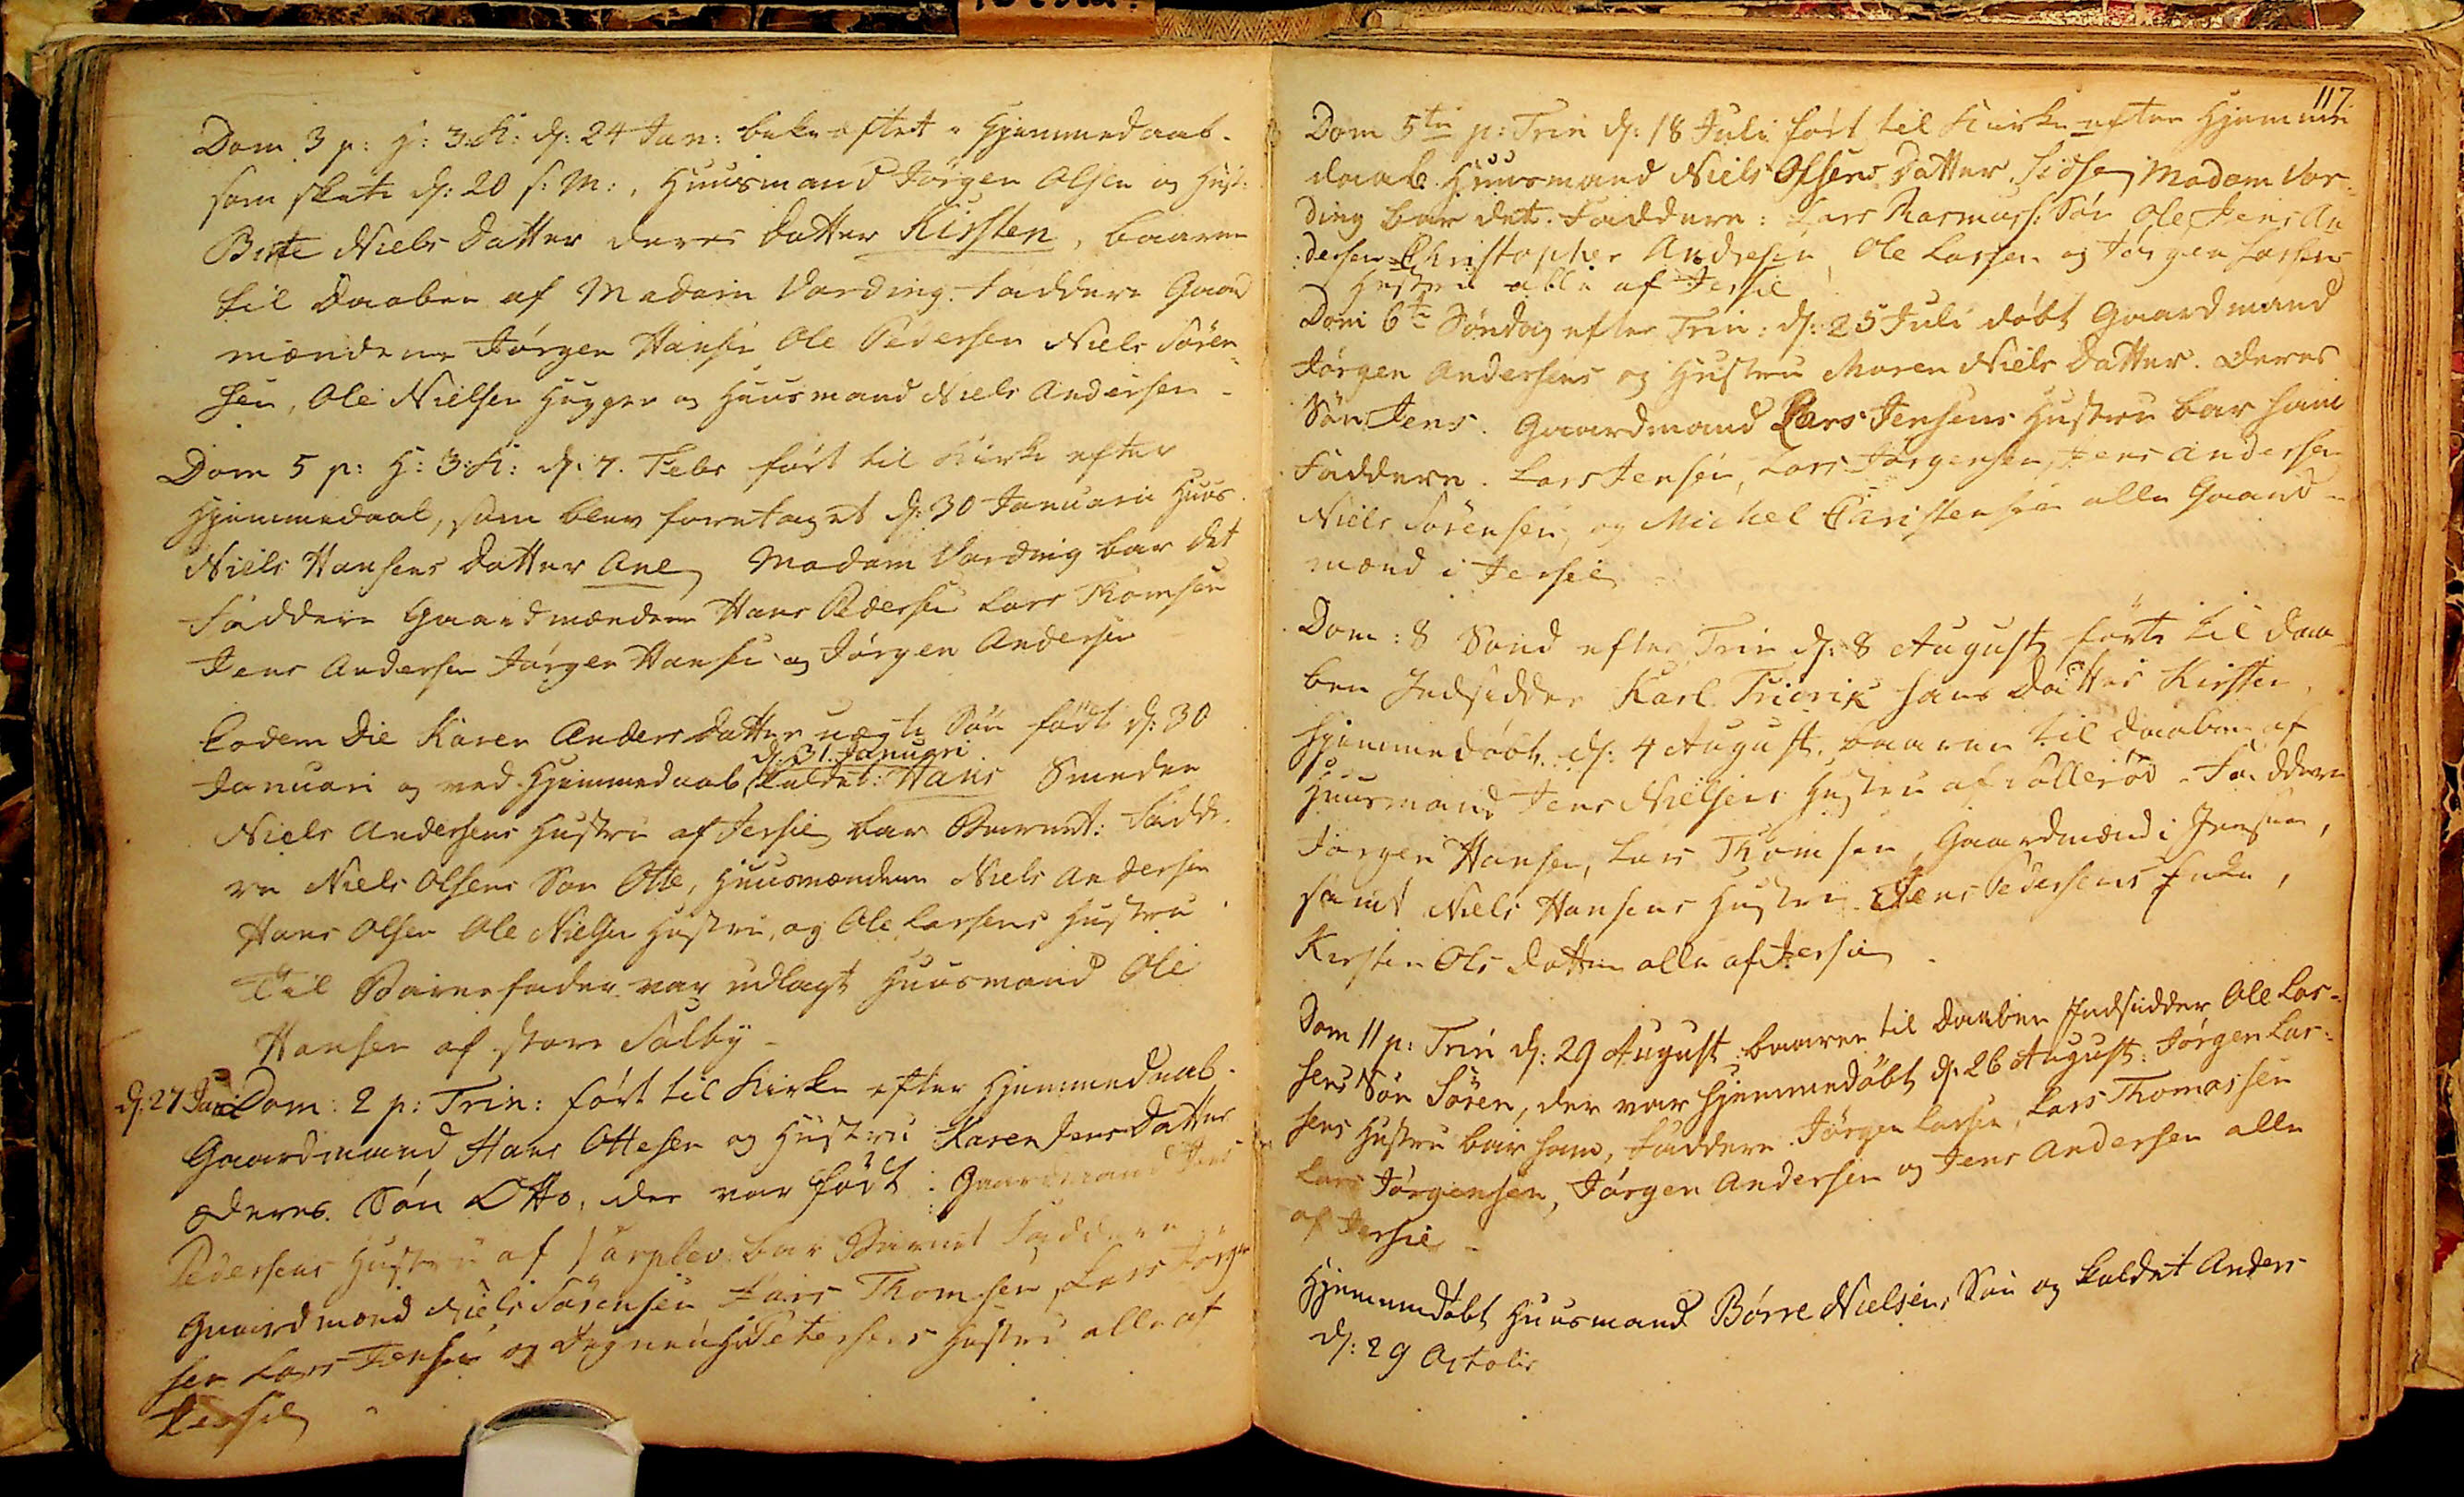Dom. 3 p: H: 3: K: d: 24 Jan: bekræftet e Hjemmedaab,
som skete d: 20 s: m:, Huusmand Jørgen Olsen og Hust:
Berte Niels Datter deres Datter Kirsten, baaren
til Daaben af Madam Vording. Faddere Gaard
mændene Jørgen Hansen Ole Pedersen Niels Søren¬
sen, Ole Nielsen Hugger og Huusmand Niels Andersen.
Dom 5 p: H: 3: K: d: 7. Febr ført til Kirke efter
Hjemmedaab, som blev foretaget d: 30 Januari Huus.
Niels Hansens Datter Ane Madam Vording bar det
Faddere Gaardmændene Hans Pedersen Lars Thomsen
Jens Andersen Jørgen Hansen og Jørgen Andersen
Eodem Die Karen Anders Datter uægte Søn født d: 30
d: 31. Januari
Januari og ved. Hjemmedaab kaldet: Hans Smeden
Niels Andersens Hustru af Jersie bar Barnet: Fadde¬
re Niels Olsens Søn Otte, Huusmændene Niels Andersen
Hans Olsen Ole Nielsens Hustru, og Ole Larsens Hustru
Til Barnefader var udlagt Huusmand Ole
Hansen af Store Salby
d: 27 Jun: Dom: 2 p: trin: ført til Kirke efter Hjemmedaab
Gaardmand Hans Ottesen og Hustru Karen Jens Datter
deres Søn Otto, der var født. Gaardmand Jens
Pedersens Hustru af Varplev: bar Barnet Faddere
Gaardmand Niels Sørensen Hans Thomsen, Lars Jørgen
sen. Lars Jensen og Degnen Hr Petersens Hustru alle af
Jersie.
117.
Dom 5te p: Trin d: 18 Juli ført til Kirke efter Hjemme
daab Huusmand Niels Olsens Datter Sidse Madam Vor¬
ding bar det. Faddere: Lars Rasmuss: Søn Ole, Jens An
dersen Christopher Andresen, Ole Larsen og Jørgen Larsens
Hustrue alle af Jersie
Dom 6te Søndag efter Trin: d: 25 Juli døbt Gaardmand
Jørgen Andersens og Hustru Maren Niels Datter. Deres
Søn Jens. Gaardmand Lars Jensens Hustru bar ham
Faddere. Lars Jensen, Lars Jørgensen, Jens Andersen
Niels Sørensen, og Michel Christensen alle Gaard¬
mænd i Jersie
Dom: 8 Sønd efter Trin d: 8 August ført til Daa¬
ben Indsidder Karl Fridrik Hans Datter Kirsten,
hjemmedøbt d: 4 August, baaren til Daaben af
Huusmand Jens Nielsens Hustru af Sollerød. Faddere
Jørgen Hansen, Lars Thomsen Gaardmænd i Jersie,
samt Niels Hansens Hustru. Jens Pedersens Enke,
Kirsten Ols Datter alle af Jersie.
Dom 11 p: Trin d: 29 August baaren til Daaben Indsidder Ole Lar¬
sens Søn Søren, der var hjemmedøbt d: 26 August: Jørgen Lar¬
sens Hustru bar ham, Faddere. Jørgen Larsen, Lars Thomassen
Lars Jørgensen, Jørgen Andersen og Jens Andersen alle
af Jersie 
Hjemmedøbt Huusmand Børre Nielsens Søn og kaldet Anders
d: 29 Octobr 
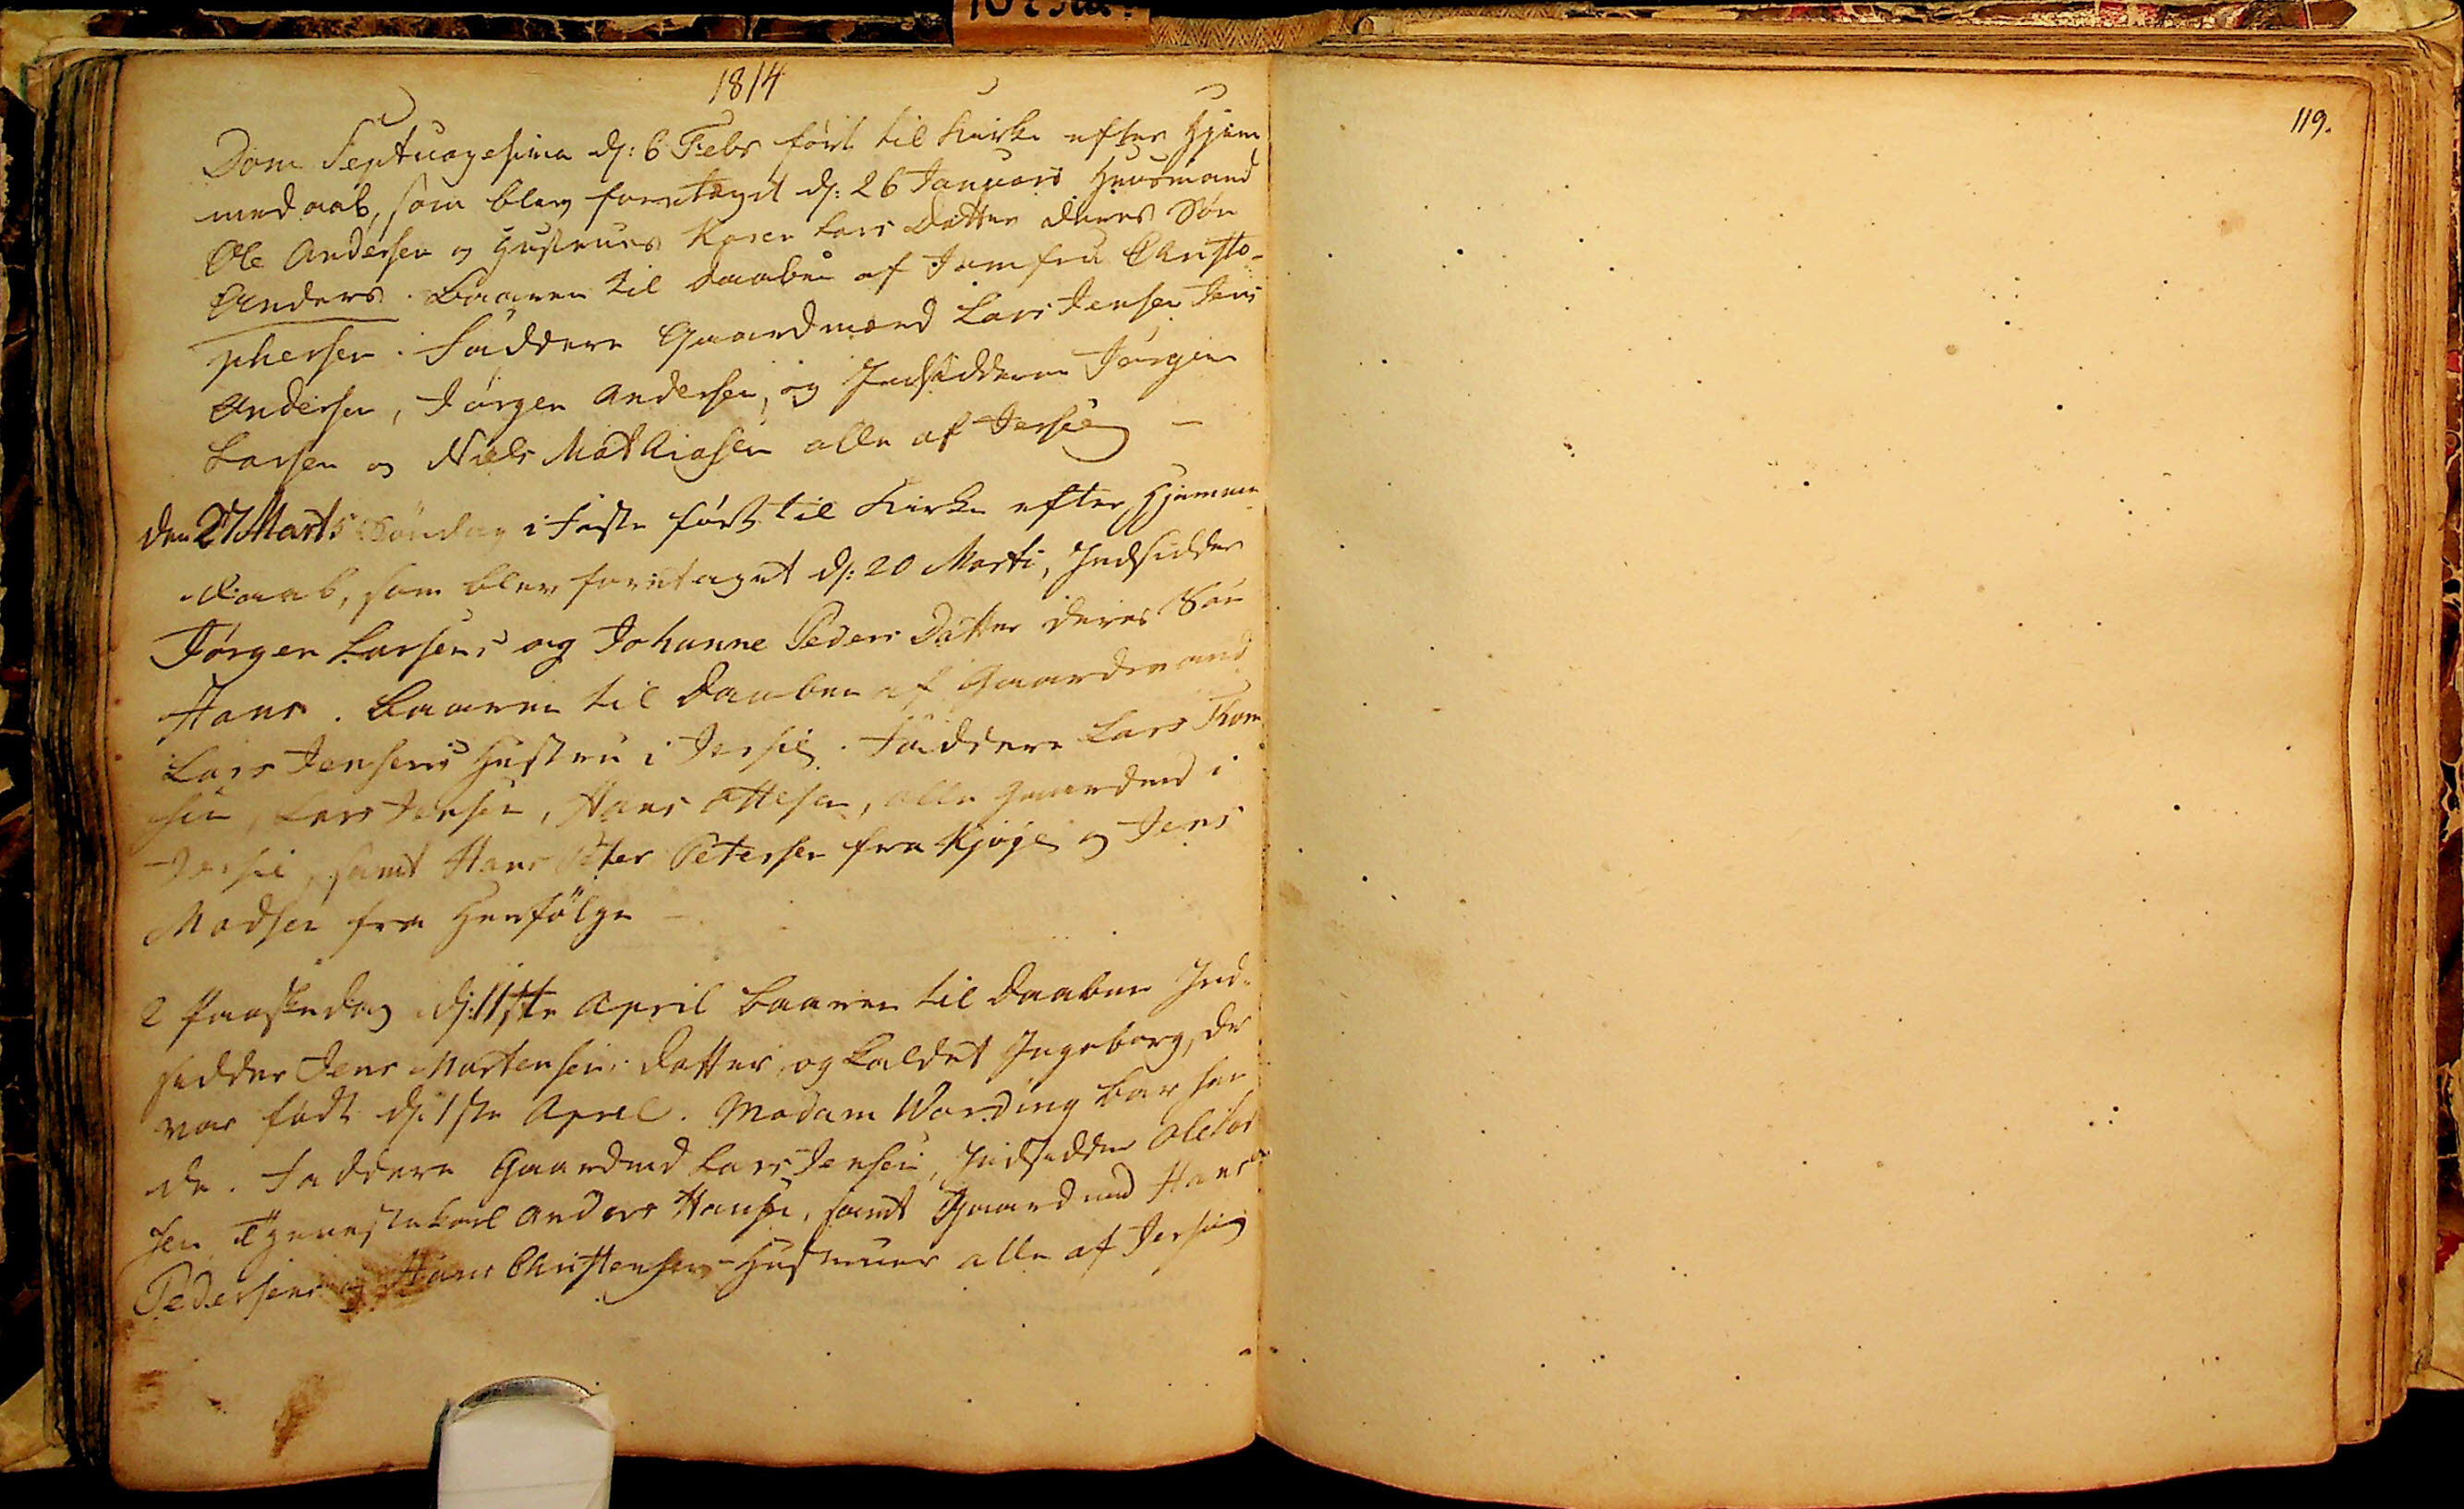1814
Dom. Septuagesima d: 6 Febr ført til Kirke efter Hjem
medaab, som blev foretaget d: 26 Januari Huusmand
Ole Andersen og Hustrues Karen Lars Datter deres Søn
Anders. Baaren til Daaben af Jomfru Christo¬
phersen. Faddere Gaardmænd Lars Jensen Jens
Andersen, Jørgen Andersen, og Indsidderne Jørgen
Larsen og Niels Mathiasen alle af Jersie -
den 27 Mart 5: Søndag i Faste ført til Kirke efter Hjemme
-daab, som blev foretaget d: 20 Marti, Indsidder
Jørgen Larsens og Johanne Peders Datter deres Søn
Hans. Baaren til Daaben af gaardmand
Lars Jensens Hustrue i Jersie, Faddere Lars Thom
sen, Lars Jensen, Hans Ottesen, alle Gaardmd i
Jersie, samt Hans Peter Petersen fra Kjøge og Jens
Madsen fra Herfølge
2 Paaskedag d 11fte April baaren til Daaben Ind¬
sider Jens Mortensens Datter, og kaldet Ingeborg, der
var født d: 1ste April. Madam Wording bar hen
de. Faddere Gaardmd Lars Jensen, Indsidder Ole Lar
sen, Tjenestekarl Anders Hansen, samt Gaardmd Hans
Pedersens og Hans Christensens Hustruer alle af Jersie
119.